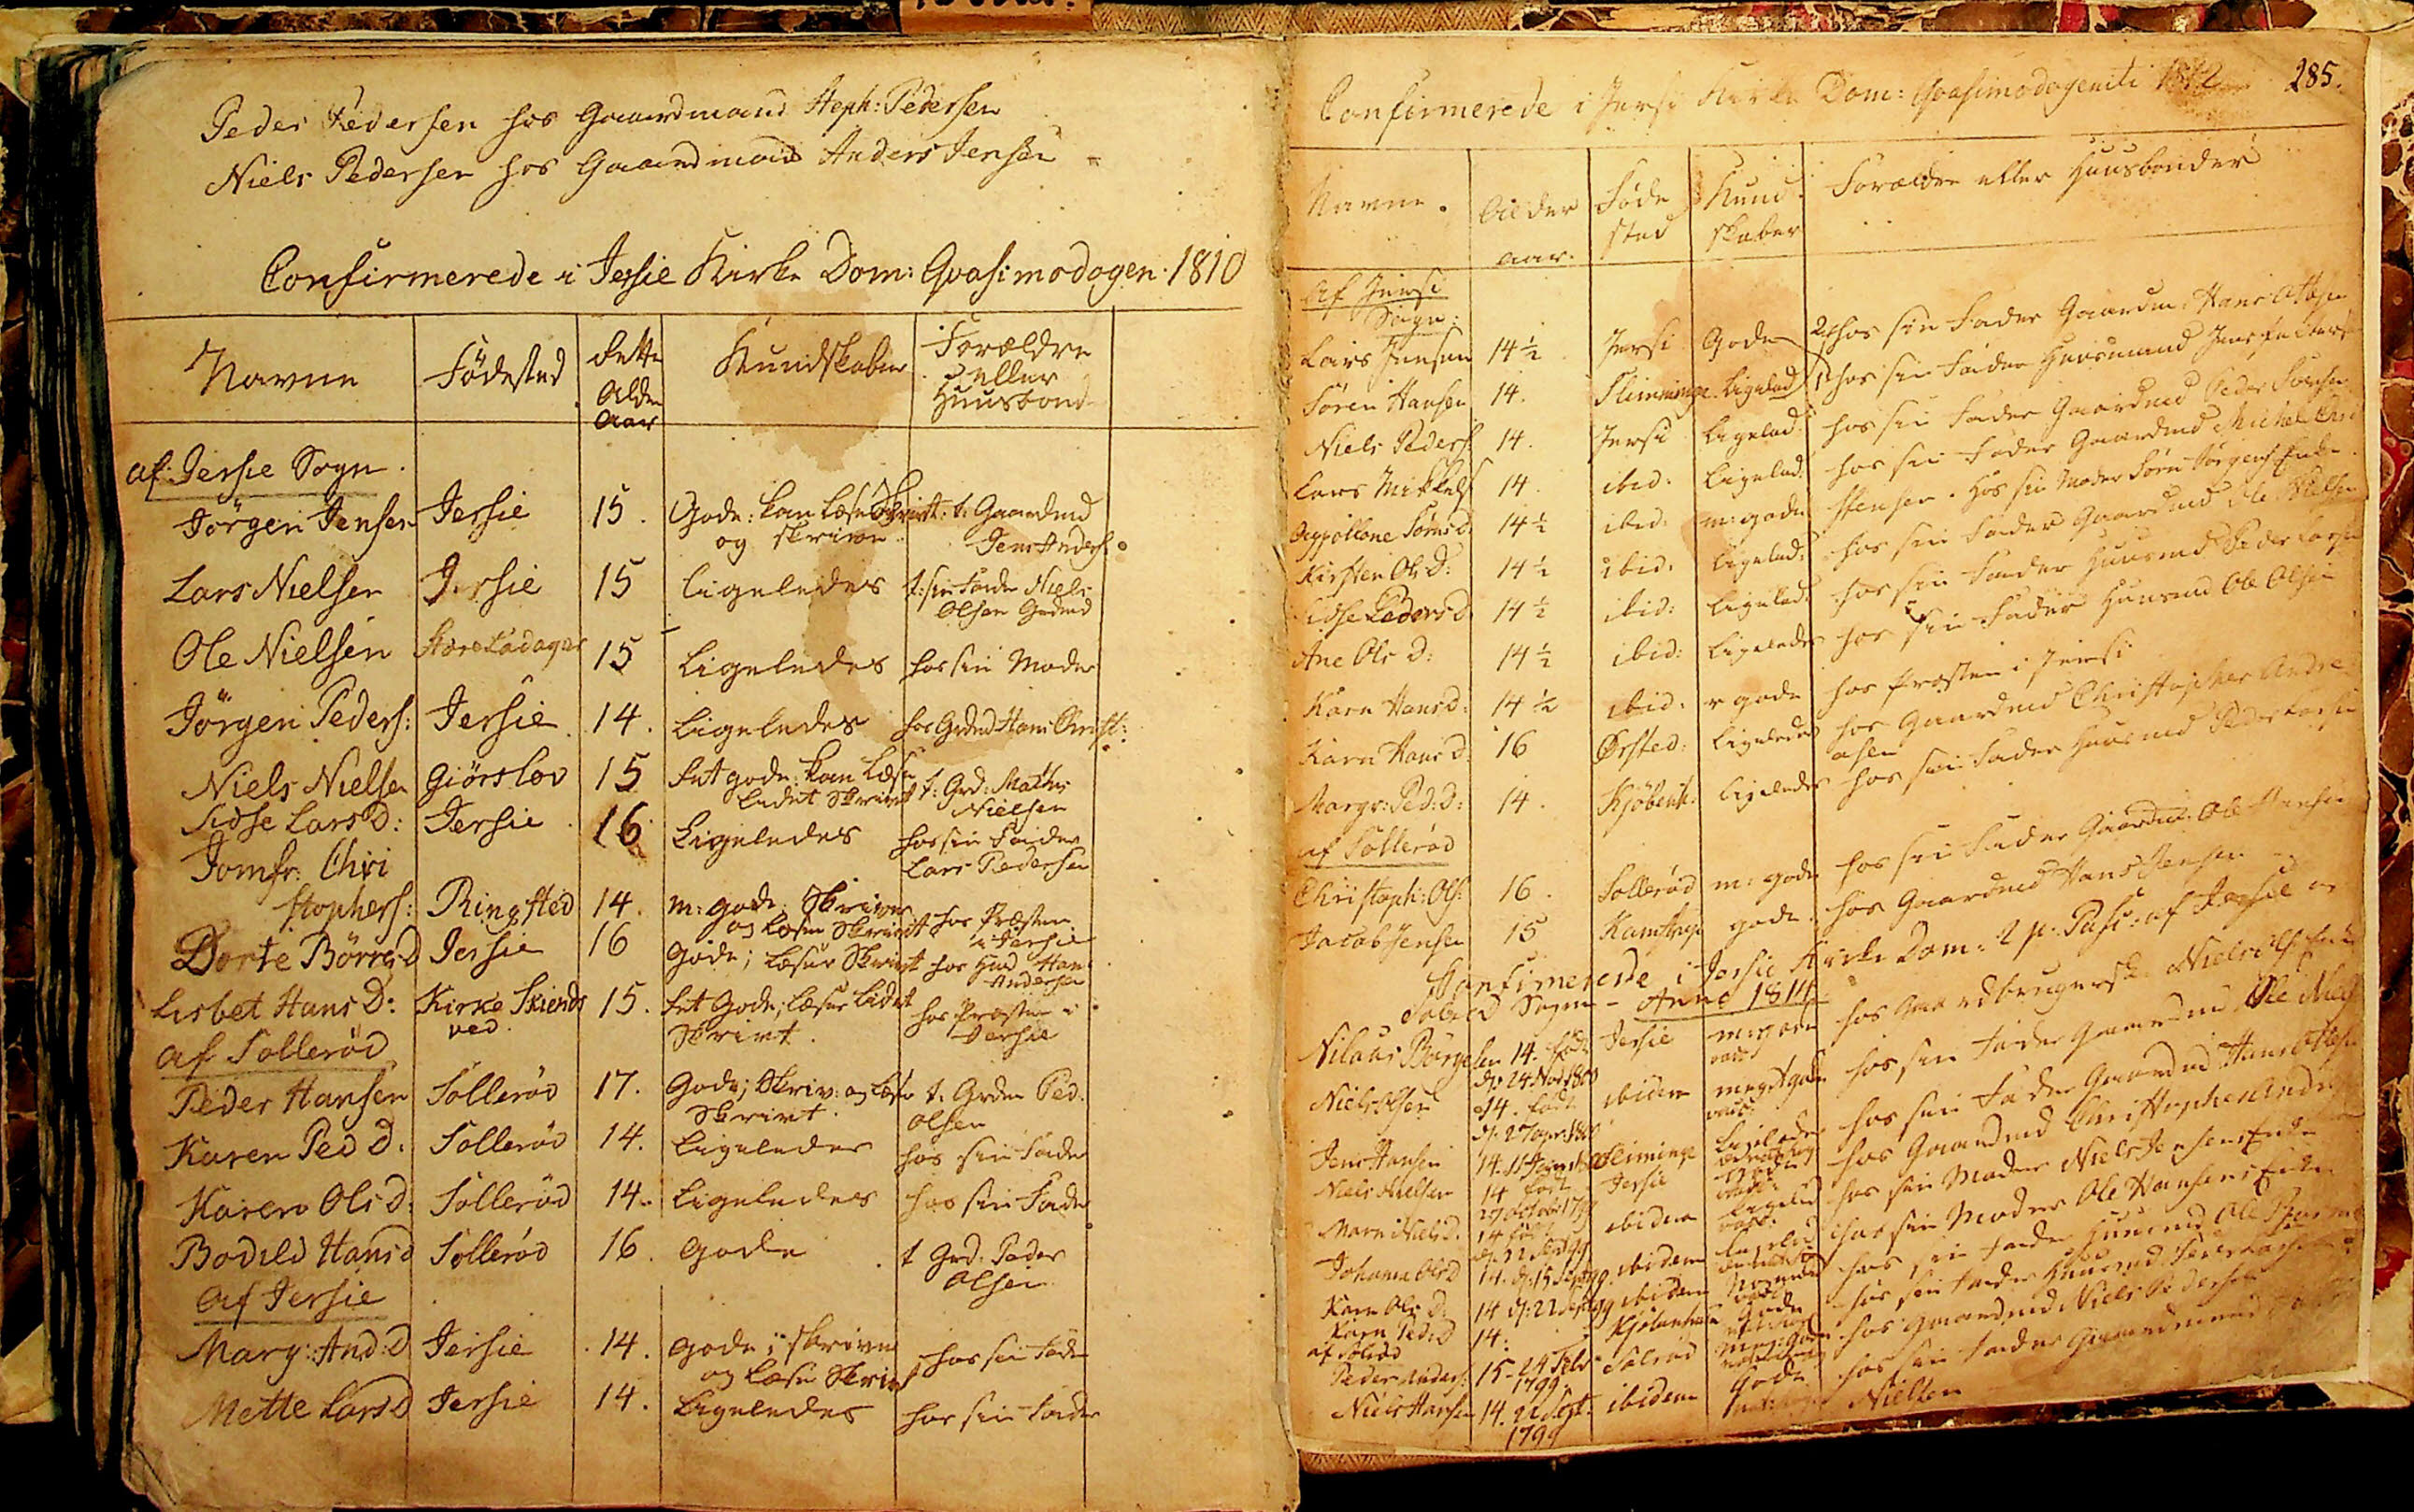Peder Pedersen hos Gaardmand Steph: Pedersen
Niels Pedersen hos Gaardmand Anders Jensen
Confirmerede i Jersie Kirke Dom: Qvasimodogen: 1810
Navne
Fødested
dette
Alder
Aar
Kundskaber
Forældre
eller
Huusbond
af Jersie Sogn
Jørgen Jensen 
Jersie
15
Gode: Kan læse Skrivt
og skrive
t: Gaardmd
Jens Anders:
Lars Nielsen
Jersie
15
ligeledes
t: sin Fader Niels
Olsen Grdmd
Ole Nielsen
Store Ladager 
15    
ligeledes      
hos sin Moder
Jørgen Peders:
Jersie    
14.   
ligeledes      
hos Grdnd. Hans Christ:                                    
Niels Nielsen
Giørsløv   
15  
Ret gode: Kan Læse
lidet Skrivt
t: Grd: Mathis
Nielsen
Sidse Larsd:
Jersie
16
ligeledes
hos sin Fader
Lars Pedersen
Jomfr: Chri
stophers:
Ringsted
14
m: gode: Skriver
og læser Skrivt
hos Præsten
i Jersie
Dorte Børre D
Jersie    
16  
Gode; Læser Skrivt   
hos Hmd Hans
Andersen
Lisbet Hans D:
af Sollerød
Kirke Skiends
ved
15.
Ret gode; læser lidet
Skrivt.
hos Præsten i
Jersie
Peder Hansen
Sollerød   
17.  
Gode; Skriver og Læser 
Skrivt.
t: Grdm Ped:
Olsen
Karen Ped D:
Sollerød
14.
ligeledes      
hos sin Fader
Karen Ols D:
Sollerød
14
ligeledes
hos sin Fader
Bodild Hans D
Af Jersie
Sollerød
16.
Gode
t Grd: Peder
Olsen
Marg: And: D
Jersie
14.
Gode; skriver     
og læser Skrivt
hos sin Fader
Mette LarsD
Jersie
14.
ligeledes
hos sin Fader
285.
Confirmerede i Jersi Kirke Dom: Qvasimodogeniti 1812
Navne.
Alder
Aar.
Føde
sted
Kund
skaber
Forældre eller Huusbonde
af Jersi
Sogn:
Lars Jensen
14½
Jersi
Gode
2 hos sin Fader Gaardm: Hans Ottesen
Søren Hansen
14.  
Slimminge 
ligeled
1 hos sin Fader Huusmand Jens Peitersen
Niels Peders:
14.
Jersi.
ligeled.
hos sin Fader Gaardmd Peder Svensen
Lars Mikkels
14
ibid:
ligeled.
hos sin Fader Gaardmd Michel Chri
Appollone Sørns D:
14 ½
ibid:
m: Gode
stensen. Hos sin Moder Sørn Jørgens Enke
Kirsten Ols D:
14 ½
ibid:
ligeled:
hos sin Fader Gaardmd Ole Nielsen
Sidse Peders D:
14 ½
ibid:
ligeled.
hos sin Fader Huusmd Peder Larsen
Ane Ols D:
14 ½
ibid:   
ligeledes
hos sin Fader Huusmd Ole Olsen
Karn Hans D:  
14 ½
ibid
r gode
hos Præsten i Jersi
Karn Hans D:
16
Ørsted:  
ligeledes
hos Gaardmd Christopher Andre
asen
Margr: Ped: D:
af Sollerød
14
Kjøbenh:
ligeledes
hos sin Fader Huusmand Peder Larsen
Christoph: Ols:
16.  
Sollerød
m: gode
hos sin Fader Gaardm: Ole Hansen
Jacob Jensen
15  
Kamstrup 
gode   
hos Gaardmd Hans Jensen
Confirmerede i Jersie Kirke Dom: 2 p: Pasc: af Jersie og
Solrød Sogne - Anno 1814:
Nilaus Børgesen
14. født
d: 24 Nov. 1800
Jersie
m: gode
vacc
hos Gaardbrugerske Niels Ols: Enke
Niels Olsen
14. født
d: 27 Apr: 1800
ibidem
meget gode
vacc
hos sin Fader Gaardmd Ole Niels
Jens Hansen
14. 11 Jan 1800
Sliminge
ligeledes
de nat. kop.
hos sin Fader Gaardmd Hans Ottesen
Niels Nielsen
14 født
27 Octobr 1799
Jersie
Gode
vacc
hos Gaardmd Christopher Andresen
Marn Niels D:
14. født
d: 15 Sept 99
ibidem
ligeled
vacc
hos sin Moder Niels Jensens Enke
Johanne Ols D
14. d: 15 Sept 99
ibidem
ligeled
de nat Kop
hos sin Moder Ole Hansens Enke
Karn Ols D:
14 d: 22 Sept 99
ibidem
nogenlnd
vacc
hos sin Fader Huusmd Ole Rasm:
Karn Ped: D
af Solrød
14:
Kjøbenhavn
Gode
nat: kop
hos sin Fader Huusmd Peder Larsen
Peder Anders:
15 - 24 Febr
1799
Solrød
Meg: Gode
naturlig: kop
hos gaardmd Niels Pedersen
Niels Hansen
14. 22 Sept.
1799
ibidem
Gode
nat: kop:
hos sin Fader Gaardmand Hans
Nielsen